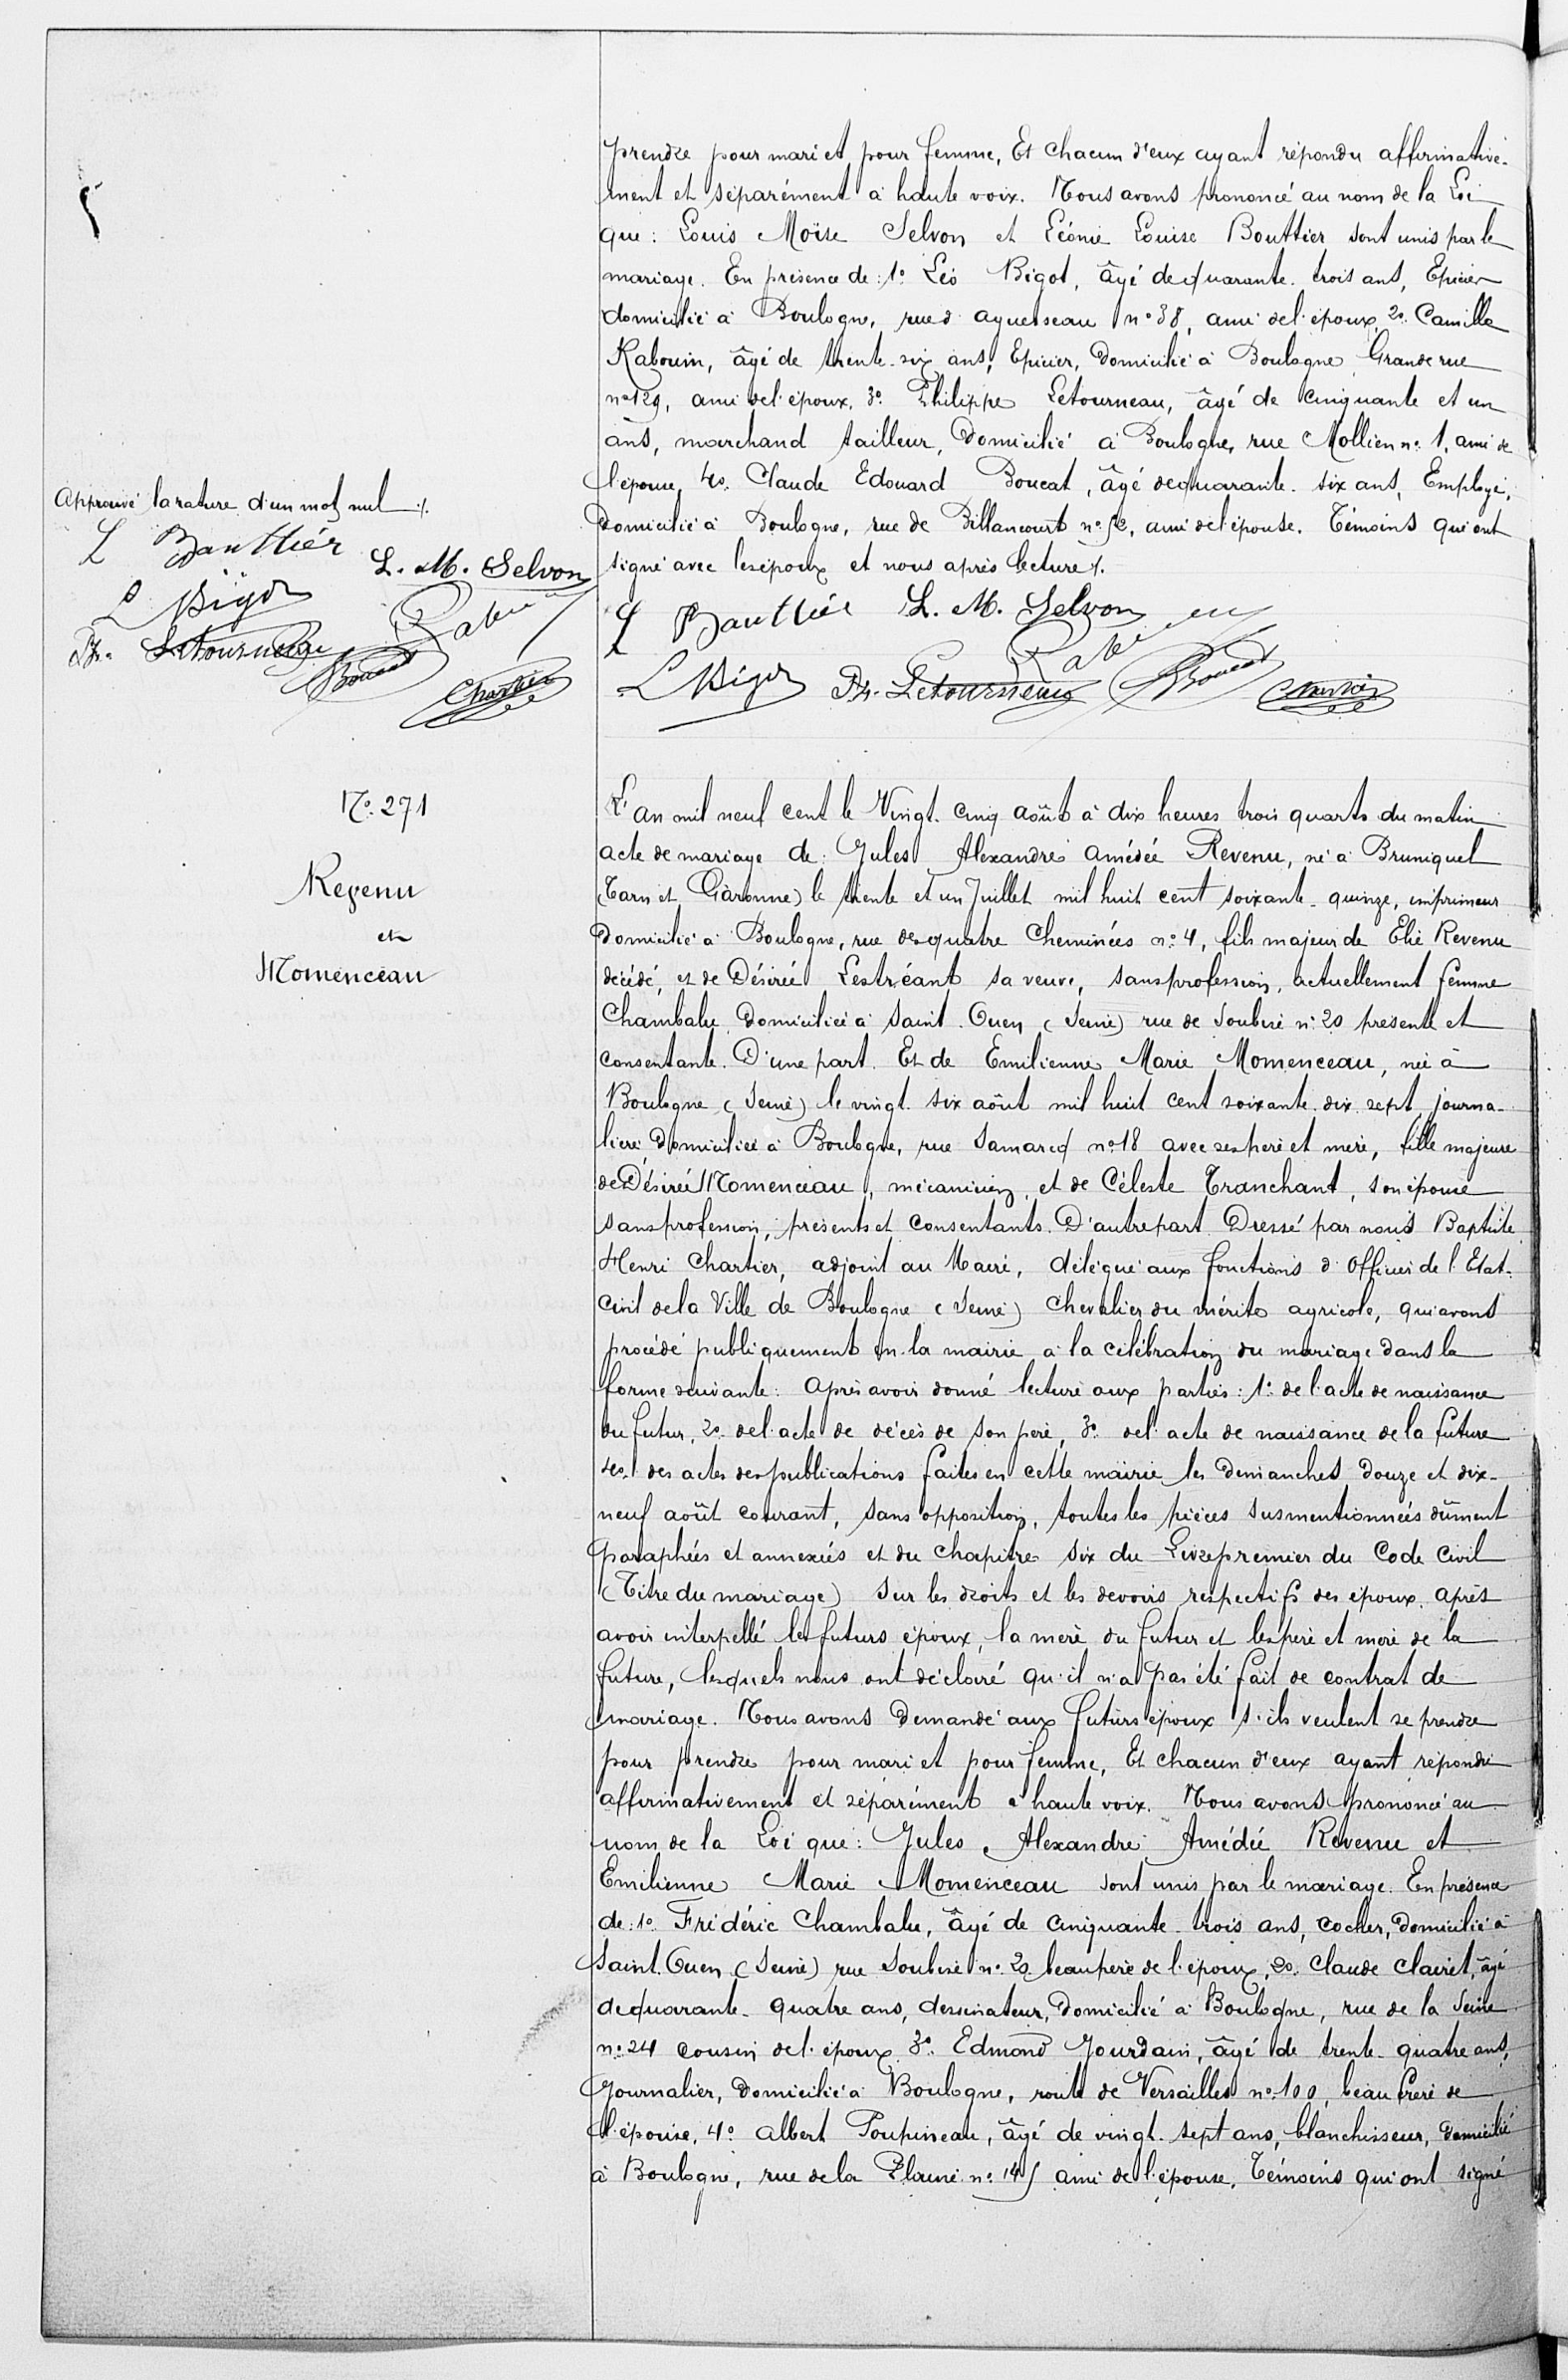prendre pour mari et pour femme, et chacun d'eux ayant répondu affirmative-
ment et séparément à haute voix. Nous avons prononcé au nom de la Loi
qui: Louis Moïse Selvon et Léonie Louise Bouttier sont unis par le
mariage. En présence de: 1° Léo Bigot, âgé de quarante trois ans, Epicier
domicilié à Boulogne, rue aguelseau n°38, ami de l'épouse 2° Camille
Rabouin, âgé de trente-six ans, Epicier, domicilié à Boulogne, Grande rue
n°129, ami de l'époux 3° Philippe Letourneau, âgé de cinquante et un
ans, marchand tailleur, domicilié à Boulogne, rue Mollien n° 1 ami de
l'épouse 4° Claude Edouard Boucat, âgé de quarante six ans, Employé,
domicilié à Boulogne, rue de Billancourt n°52, ami de l'épouse. Témoins qui ont
signé avec les époux et nous après lecture ./.
N°271
Revenu
et
Momenceau
L'an mil neuf cent le vingt cinq août à dix heures trois quarts du matin
acte de mariage de Jules Alexandre Amédée Revenu, né à Bruniquel
(Tarn et Garonne) le trente et un juillet mil huit cent soixante-quinze, imprimeur
domicilié à Boulogne, rue de quatre cheminées n°4, fils majeur de Ché Revenu
décédé, et de Désirée Lestréant sa veuve, sans profession, actuellement femme
chambalie domiciliée à saint ouen (Seine) rue de soubré n°20 présente et
consentante. D'une part. Et de Emilienne Marie Momenceau, née à
Boulogne (Seine) le vingt six août mil huit cent soixante dix sept, journa-
lière domiciliée à Boulogne, rue samarid n°18 avec ses père et mère, fille majeure
de Désirée Momenceau, mécanicien, et de Céleste Tranchard, son épouse
sans profession, présents et consentants. D'autre part. Dressé par nous Baptiste
Henri Chartier, adjoint au Maire, délégué aux fonctions d'officier de l'Etat
Civil de la Ville de Boulogne (Seine) Chevalier du mérite agricole, qui avons
procédé publiquement en la mairie à la célébration du mariage dans la
forme suivante: après avoir donné lecture aux parties: 1° de l'acte de naissance
du futur, 2° de l'acte de décès de son frère, 3° de l'acte de naissance de la future
4° des actes des publications faites en cette mairie les dimanches douze et dix-
neuf août courant, sans opposition, toutes les pièces susmentionnées dûment
paraphées et annexées et du chapitre six du livre premier du Code Civil
(Titre du mariage) sur les droits et les devoirs respectifs des époux après
avoir interpellé les futurs époux, la mère du futur et le père et mère de la
future, lesquels nous ont déclaré qu'il n'a pas été fait de contrat de
mariage. Nous avons demandé aux futurs époux s'ils veulent se prendre
pour prendre pour mari et pour femme, et chacun d'eux ayant répondu
affirmativement et séparément à haute voix. Nous avons prononcé au
nom de la Loi que: Jules Alexandre Amédée Revenu et
Emilienne Marie Momenceau sont unis par le mariage. En présence
de 1° Frédéric Chambalu, âgé de cinquante trois ans cocher domicilié à
Saint Ouen (Seine) rue soubire n° 20 beau père de l'époux, 2° Claude Clairet, âgé
de quarante-quatre ans, dessinateur, domicilié à Boulogne, rue de la Seine
n° 24 cousin de l'épouse 3° Edmond Jourdain âgé de trente quatre ans
journalier domicilié à Boulogne, route de Versailles n°100, beau frère de
l'épouse, 4° Albert Poupineau, âgé de vingt-sept ans, blanchisseur, domicilié
à Boulogne, rue de la Plaine n°145 ami de l'épouse, témoins qui ont signé
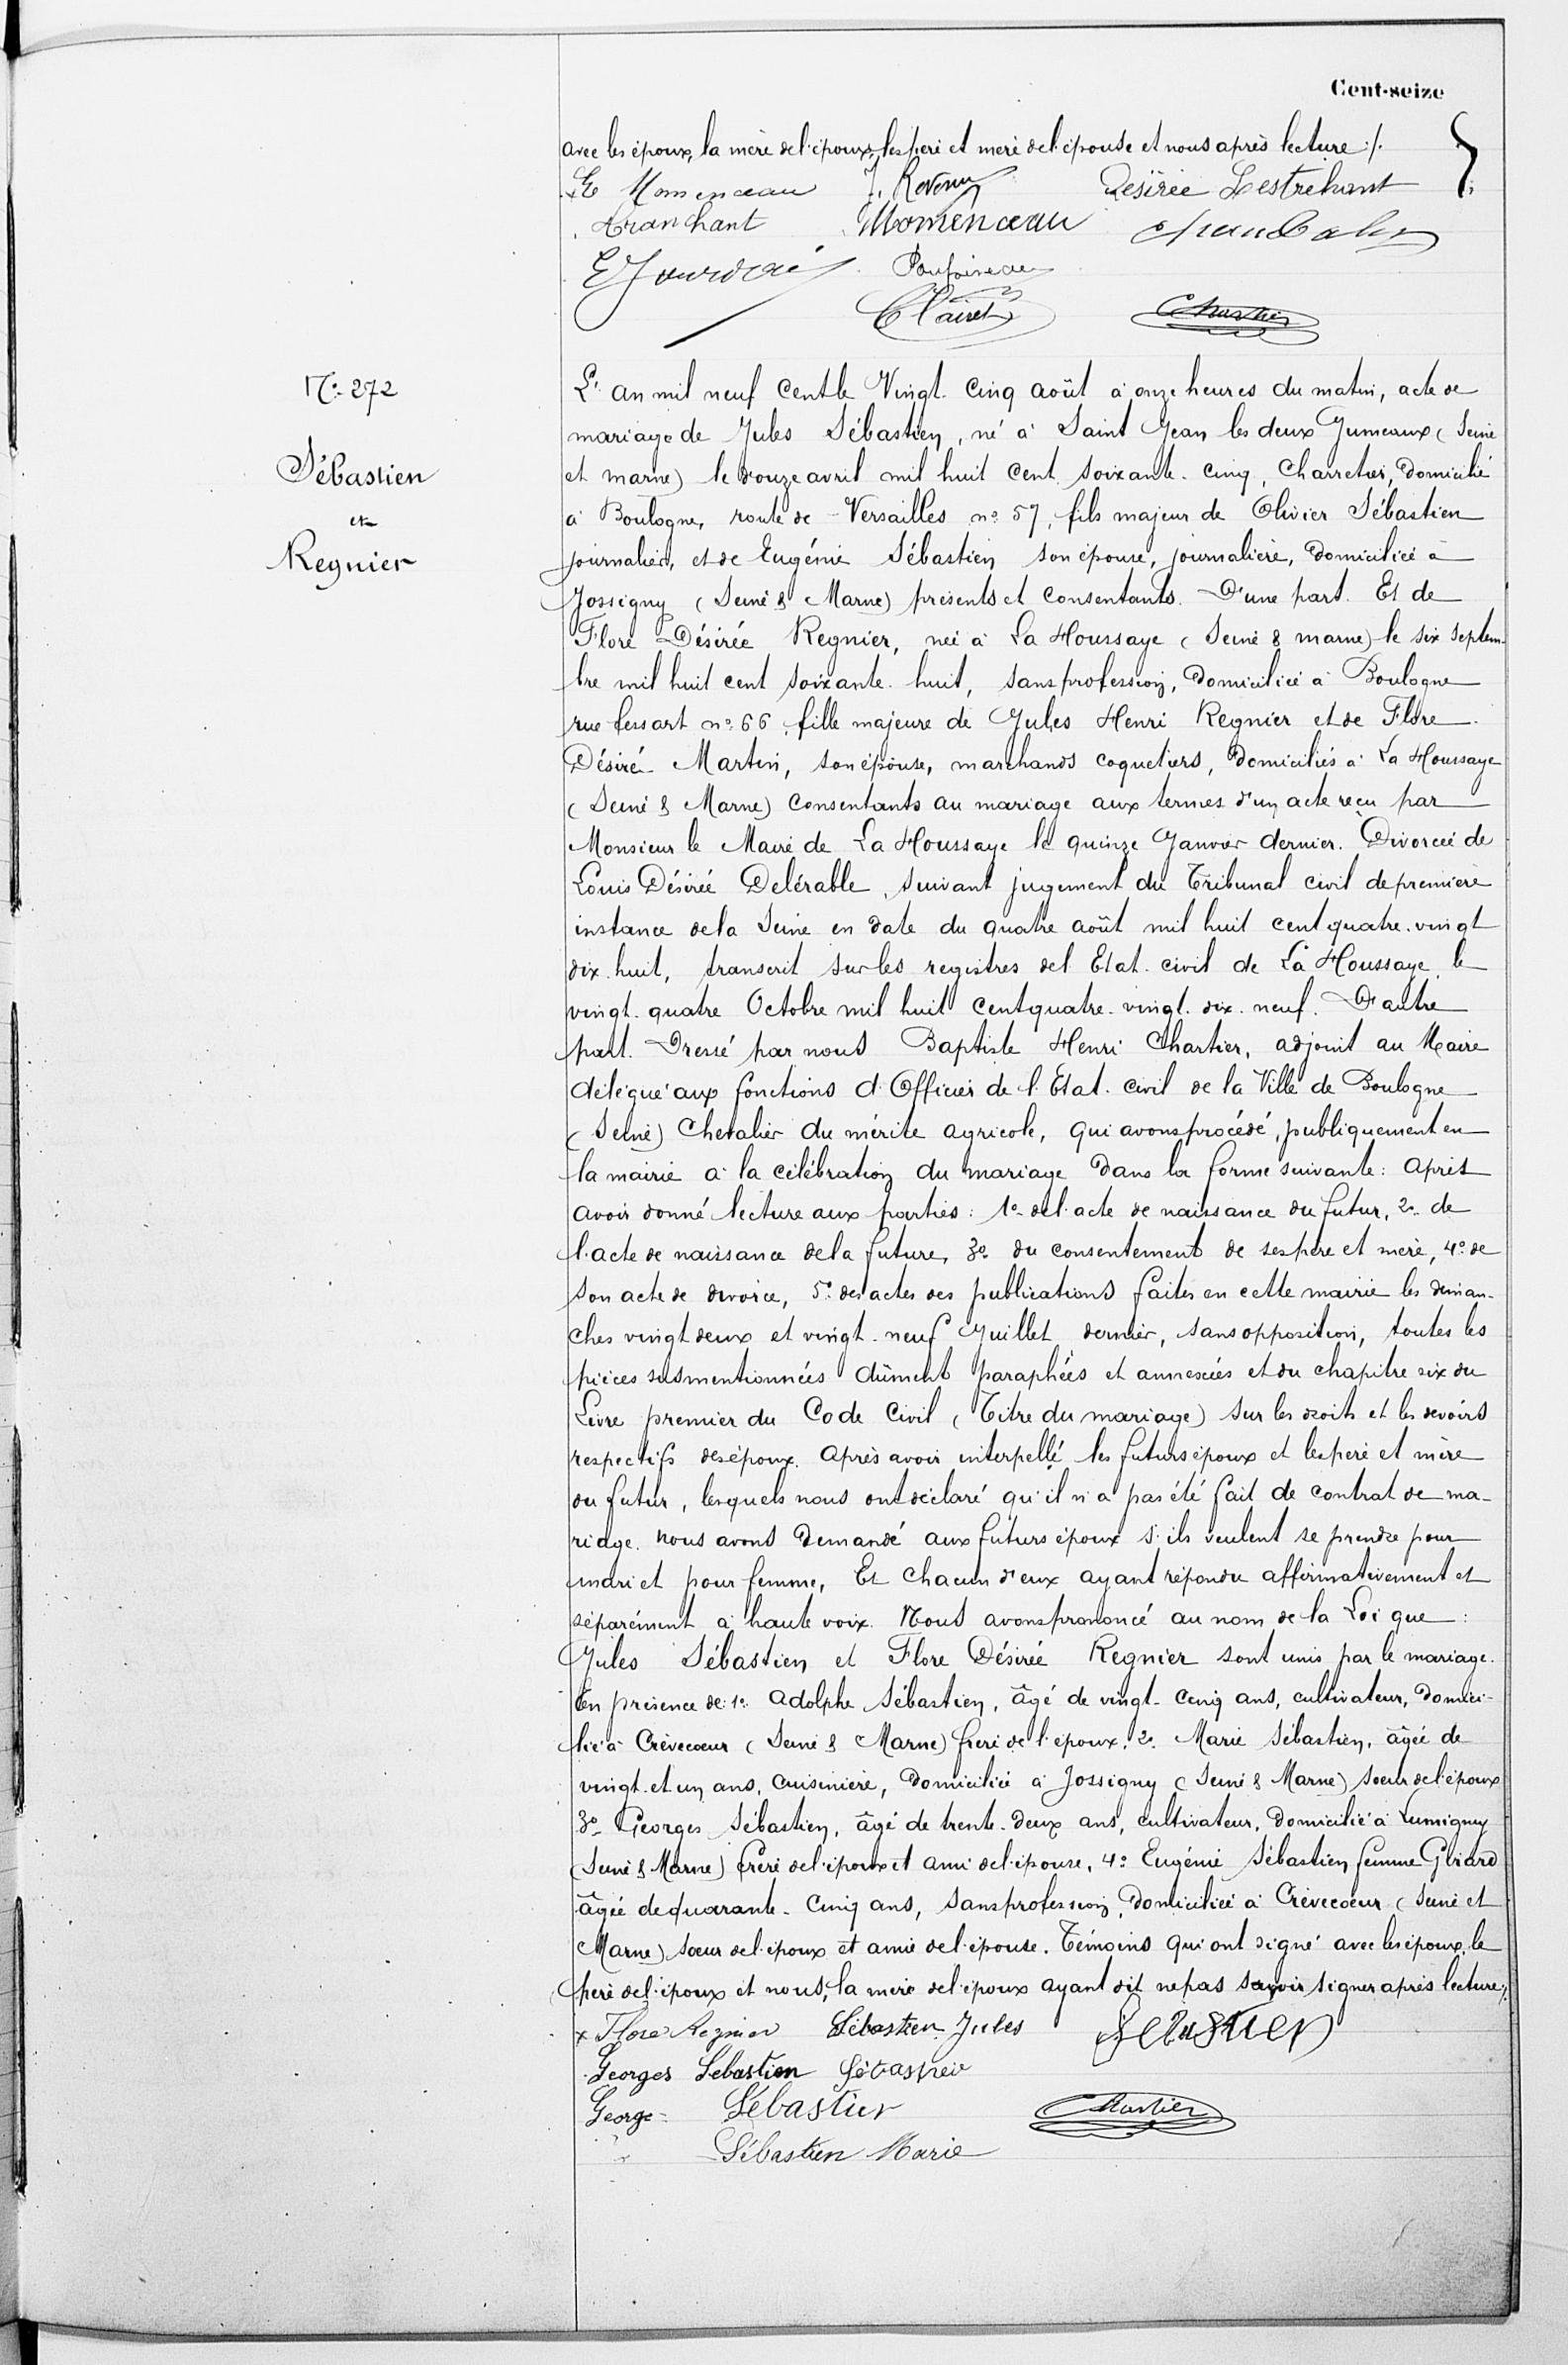avec les époux, la mère de l'époux, les père et mère de l'épouse et nous après lecture ./.
N° 272
Sébastien
et Regnier
l'an mil neuf cent le vingt cinq août à onze heures du matin, acte de
mariage de Jules Sébastien, né à Saint Jean les deux jumeaux (Seine
et Marne) le douze avril mil huit cent soixante-cinq, charretier, domicilié
à Boulogne, route de Versailles n°57, fils majeur de Olivier Sébastien
journalier, et de Eugénie Sébastien son épouse, journalière, domiciliée à
Jossigny (Seine et Marne) présents et consentants. D'une part. Et de
Flore Désirée Regnier, née à la Hoursaye (Seine et Marne) le six septem
bre mil huit cent soixante huit, sans profession, domiciliée à Boulogne
rue les art n°66, fille majeure de Jules Henri Regnier et de Flore
Désiré Martin, son épouse, marchands coquetiers, domiciliés à la Houssaye
(Seine et Marne) consentants au mariage aux termes d'un acte reçu par
Monsieur le Maire de la Houssaye le quinze janvier dernier. Divorcée de
Louis Désirée Delérable, suivant jugement du Tribunal civil de première
instance de la Seine en date du quatre août mil huit cent quatre vingt
dix-huit, transcrit sur les registres de l'Etat civil de la Houssaye, le
vingt-quatre octobre mil huit cent quatre vingt dix neuf. D'autre
part. Dressé par nous Baptiste Henri Chartier, adjoint au Maire
délégué aux fonctions d'Officier de l'Etat civil de la Ville de Boulogne.
(Seine) Chevalier du mérite agricole, qui avons procédé, publiquement en
la mairie à la célébration du mariage dans la forme suivante: après
avoir donné lecture aux parties: 1° de l'acte de naissance du futur, 2° de
l'acte de naissance de la future, 3° du consentement de ses père et mère, 4° de
son acte de service, 5° des actes des publications faites en cette mairie les diman
ches vingt deux et vingt-neuf juillet dernier, sans opposition, toutes les
pièces susmentionnées dûment paraphées et annexées et du chapitre six du
Livre premier du Code Civil (Titre du mariage) sur les droits et les devoirs
respectifs des époux. Après avoir interpellé les futurs époux et les père et mère
du futur, lesquels nous ont déclaré qu'il n'a pas été fait de contrat de ma
riage nous avons demandé aux futurs époux s'ils veulent se prendre pour
mari et pour femme. Et chacun d'eux ayant répondu affirmativement et
séparément à haute voix nous avons prononcé au nom de la Loi que:
Jules Sébastien et Flore Désirée Regnier sont unis par le mariage.
En présence de 1°: Adolphe Sébastien, âgé de vingt-cinq ans, cultivateur, domici-
lié à Crèvecoeur (Seine et Marne) frère de l'époux 2° Marie sébastien, âgée de
vingt et un ans, cuisinière, domiciliée à Jossigny (Seine et Marne) soeur de l'époux
3° Georges Sébastien, âgé de trente-deux ans, cultivateur, domicilié à Lumigny
(Seine et Marne) frère de l'époux et ami de l'épouse, 4: Eugénie Sébastien femme Gliard
âgée de quarante-cinq ans, sans profession, domiciliée à Crèvecoeur (Seine et
Marne) soeur de l'époux et amie de l'épouse. Témoins qui ont signé avec les époux, le
frère de l'époux et nous la mère de l'époux ayant dit ne pas savoir signer après lecture